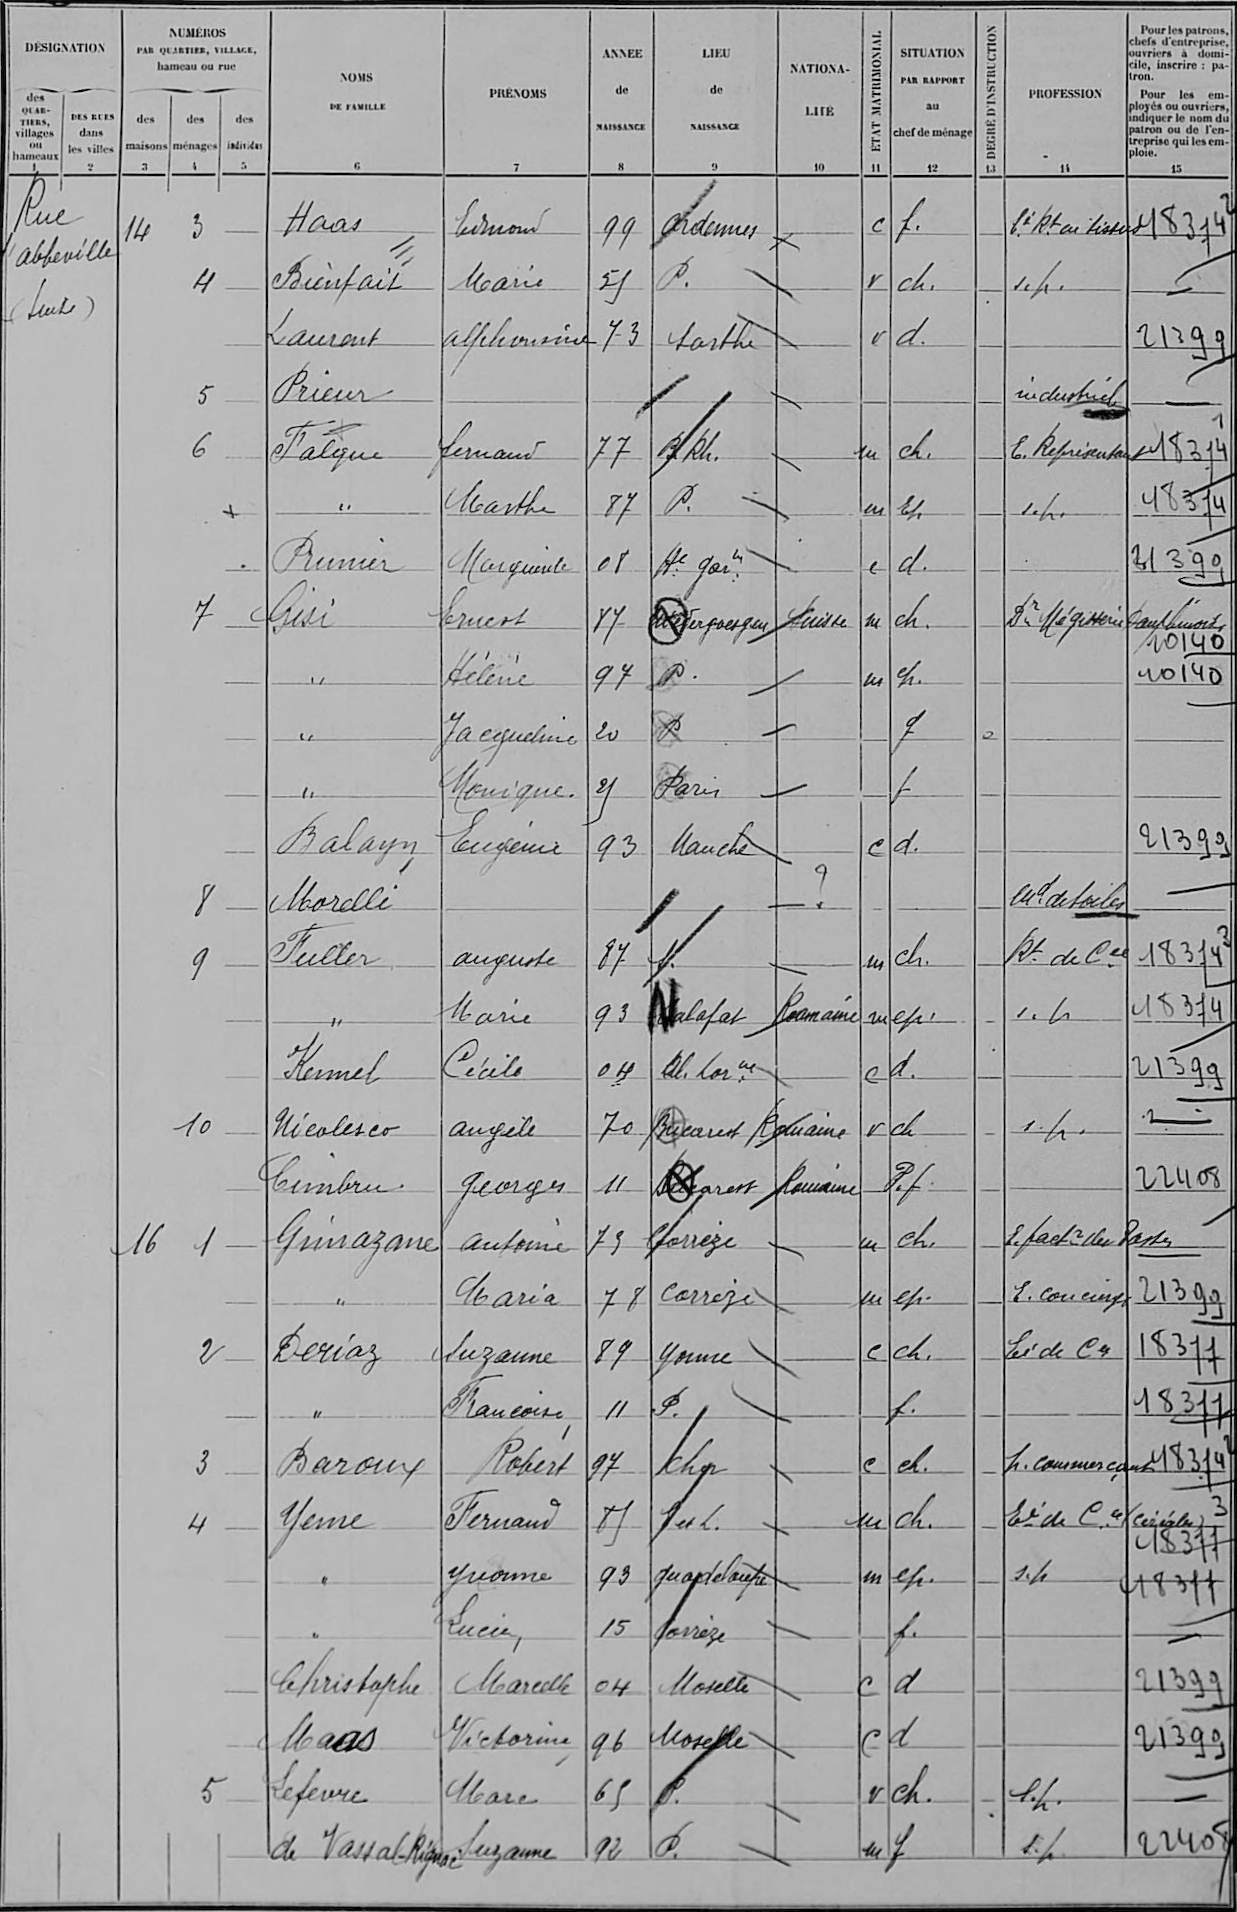Haas/Edmond/99/Ardennes/¤/c/f /¤/Eé Rt en tissus/18374
Bienfait/Marie/55/P /¤/c/ch /¤/sp /¤
Laurent/Alphonsine/73/Sarthe/¤/v/d /¤/¤/21399
Prieur/¤/¤/¤/¤/¤/¤/¤/industriel/¤
Falque/fernand/77/B Rh /¤/m/Ch /¤/E Représentant/18374
¤/Marthe/87/P /¤/m/Ep/¤/s p /18374
Prunier/Marguerite/08/Hte Garne/¤/c/d /¤/¤/21399
Gisi/Ernest/87/Niedergoesgen/Suisse/m/ch /¤/Dr Mégisserie Dauphinois/!10140
¤/Hélène/97/P/¤/m/ep/¤/¤/10140
¤/Jacqueline/20/P/¤/¤/f/o/¤/¤
¤/Monique/25/Paris/¤/¤/f/¤/¤/¤
Balayn/Eugénie/93/Manche/¤/c/d /¤/¤/21399
Morelli/¤/¤/¤/¤/¤/¤/¤/eme de toiles/¤
Fuller/augvuste/87/S /¤/m/ch /¤/Rt de Cce/18374
¤/Marie/93/Salafat/Roumanie/m/ep/¤/s p/18374
Kermel/Cécile/04/Al Lorne/¤/c/d /¤/¤/21399
Nicolesco/Angèle/70/Bucarest/Roumaine/v/ch/¤/s p /¤
Timbru/Georges/11/Bucarest/Romaine/¤/P f /¤/¤/22408
Guinazane/Antoine/73/Corrèze/¤/m/ch /¤/E factr des Postes/¤
¤/Maria/78/Corrèez/¤/m/ep /¤/E concierge/21399
Derias/Suzanne/89/Yonne/¤/C/Ch /¤/Eé de Cce/18377
¤/Françoise/11/P /¤/¤/f /¤/¤/18377
Baroux/Robert/9/Cher/¤/c/ch /¤/p commerçant/18374
Yeme/Fernand/85/S et L /¤/m/ch /¤/Eé e Cce (céréales)/!18377
¤/Yvonne/93/Guadeloupe/¤/m/ep /¤/s p/18377
¤/Lucien/15/Corrèze/¤/¤/f /¤/¤/¤
Christophe/Marcelle/04/Moselle/¤/C/d/¤/¤/21399
Maas/Victorine/96/Moselle/¤/C/d/¤/¤/21399
Lefevre/Marc/65/P /¤/v/ch /¤/s p /¤
de Vassal-Rignac/Suzanne/92/P /¤/m/f/¤/s p/22408
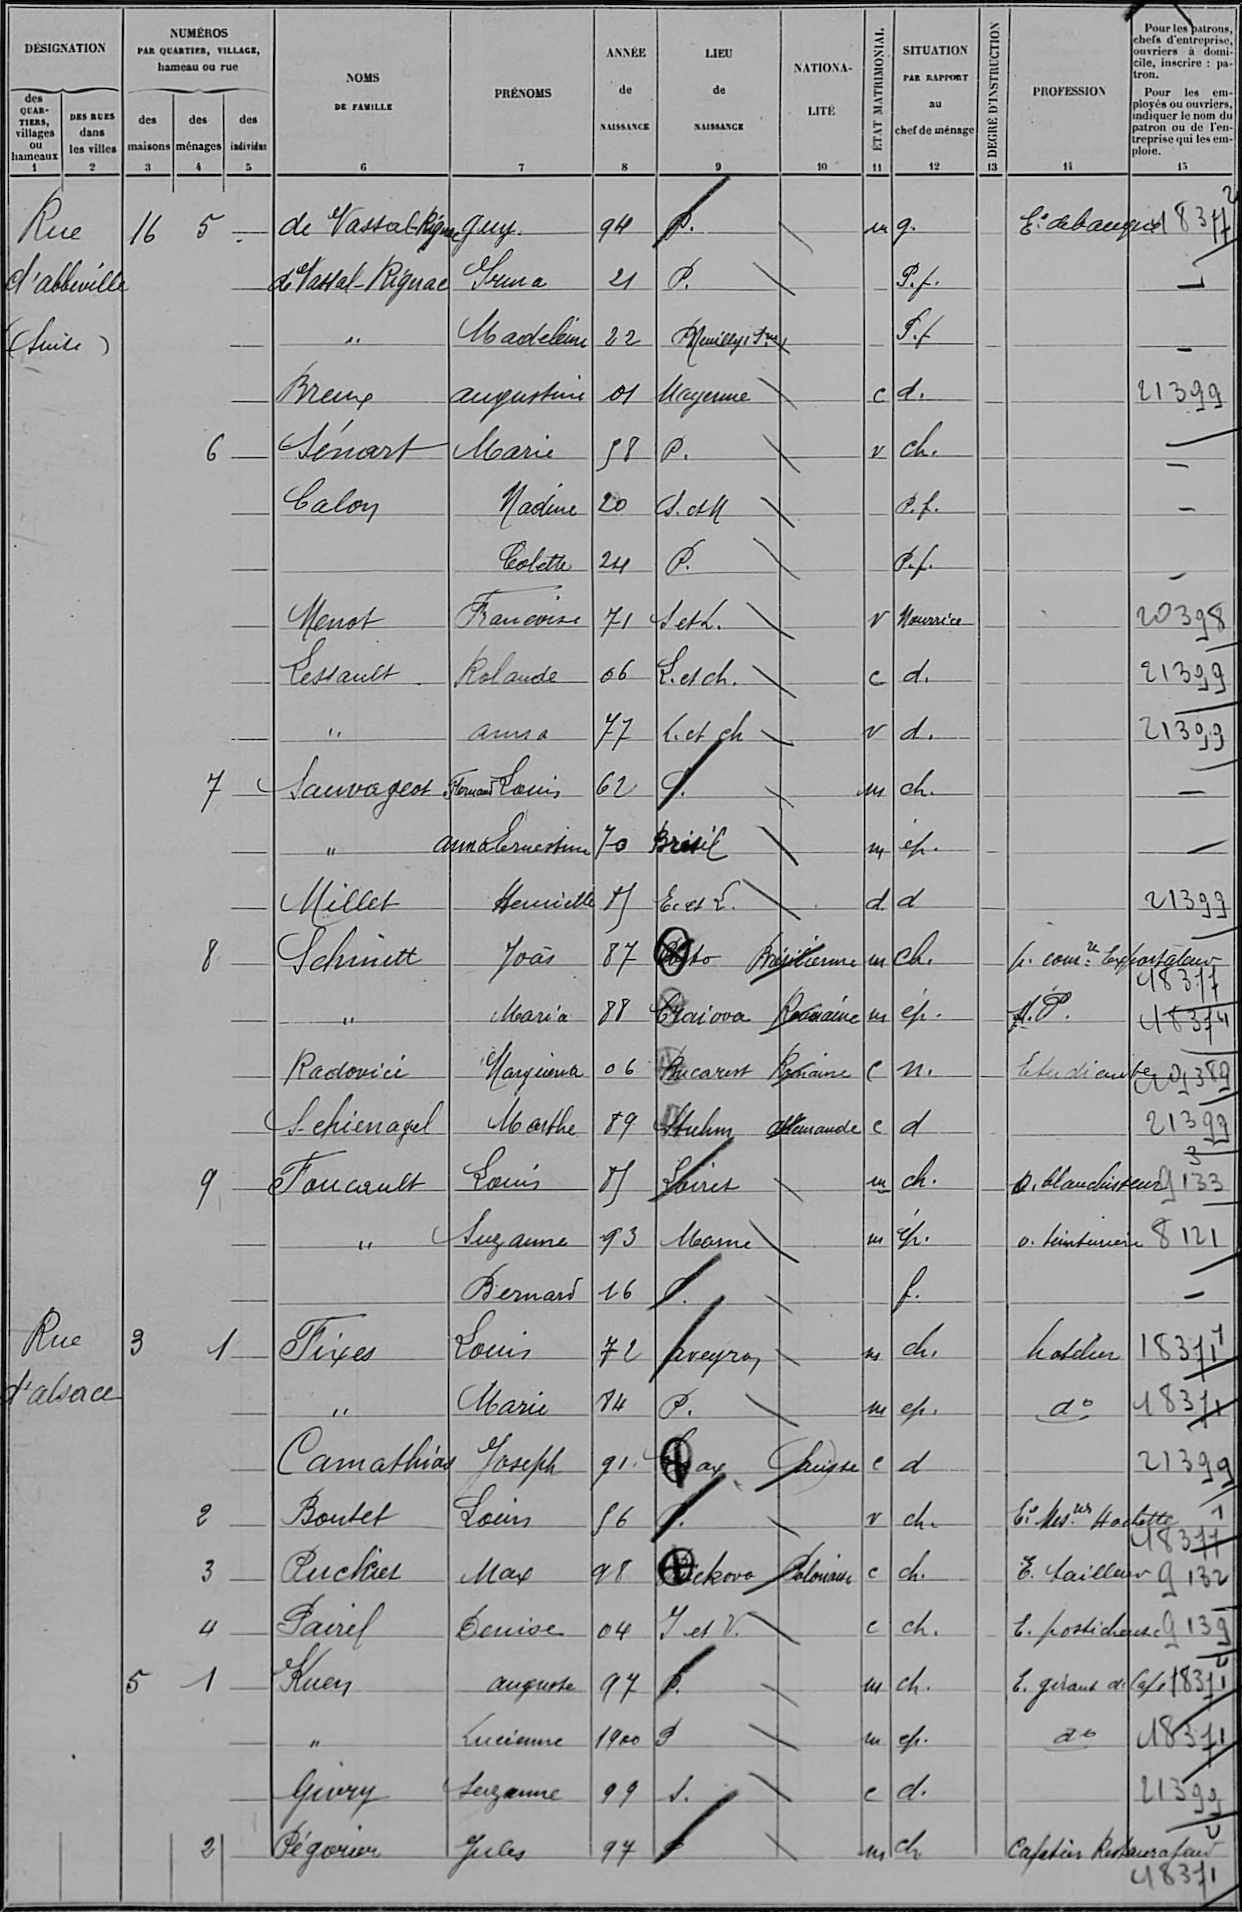de Vassal-Rignac/Guy/94/P /¤/m/f/¤/E de banque/18377
de Vassal-Rignac/Irma/21/P /¤/¤/P f /¤/¤/¤
¤/Madeleine/2/Neuilly sur Seine/¤/¤/P f /¤/¤/¤
Breux/Augustine/01/Mayenne/¤/c/d /¤/¤/21399
Sénart/Marie/58/P /¤/v/ch /¤/¤/¤
Talon/Nadine/20/S et M/¤/¤/P f /¤/¤/¤
¤/Colette/24/P /¤/¤/P f /¤/¤/¤
Menot/Françoise/71/S et L /¤/V/nourrice/¤/¤/20398
Lessault/Rolande/06/L et Ch /¤/c/d /¤/¤/21399
"/Anna/77/L et Ch/¤/v/d /¤/¤/21399
Sauvagest/Fernand Louis/62/S /¤/m/ch /¤/¤/¤
"/Anna Ernestine/70/Brésil/¤/m/ép/¤/¤/¤
Millet/Henriette/85/E et L /¤/d/d/¤/¤/21399
Schmitt/Joris/87/Bahia/Brésilienne/m/Ch /¤/p comre exporteteur/!18377
¤/MAria/88/Craiova/Roumaine/m/ép /¤/f P /18374
Radovici/Marguerite/06/Bucarest/Romaine/C/N /¤/Etudiante/19389
Schienagel/MArthe/89/Stuhm/Allemande/c/d/¤/¤/21399
Foucault/Louis/87/Loiret/¤/m/ch /¤/o blanchisseur/9133
"/Suzanne/93/Marne/¤/m/ép/¤/o teinturière/8121
¤/Bernard/16/P /¤/¤/f /¤/¤/¤
Fixes/Louis/72/aveyron/¤/m/ch /¤/hotelier/18371
¤/Marie/84/P /¤/m/ep /¤/d°/18371
Camathias/Joseph/91/Laax/Suisse/c/d/¤/¤/21399
Boutet/Louis/56/P /¤/v/ch /¤/Eé Mesre Hachette/!18377
Puckiet/Max/98/Richovo/Polonaise/c/ch /¤/E tailleur/9132
Pairel/Denise/04/I et V /¤/c/ch /¤/E posticheuse/9139
Kuen/auguste/97/P /¤/m/ch /¤/E gérant de café/18371
¤/Lucienne/1900/P/¤/m/ep /¤/d°/18371
Givry/Suzanne/99/S /¤/c/d /¤/¤/21399
Pégorien/Jules/97/P/¤/m/ch/¤/cafetier Restaurateur/!18371